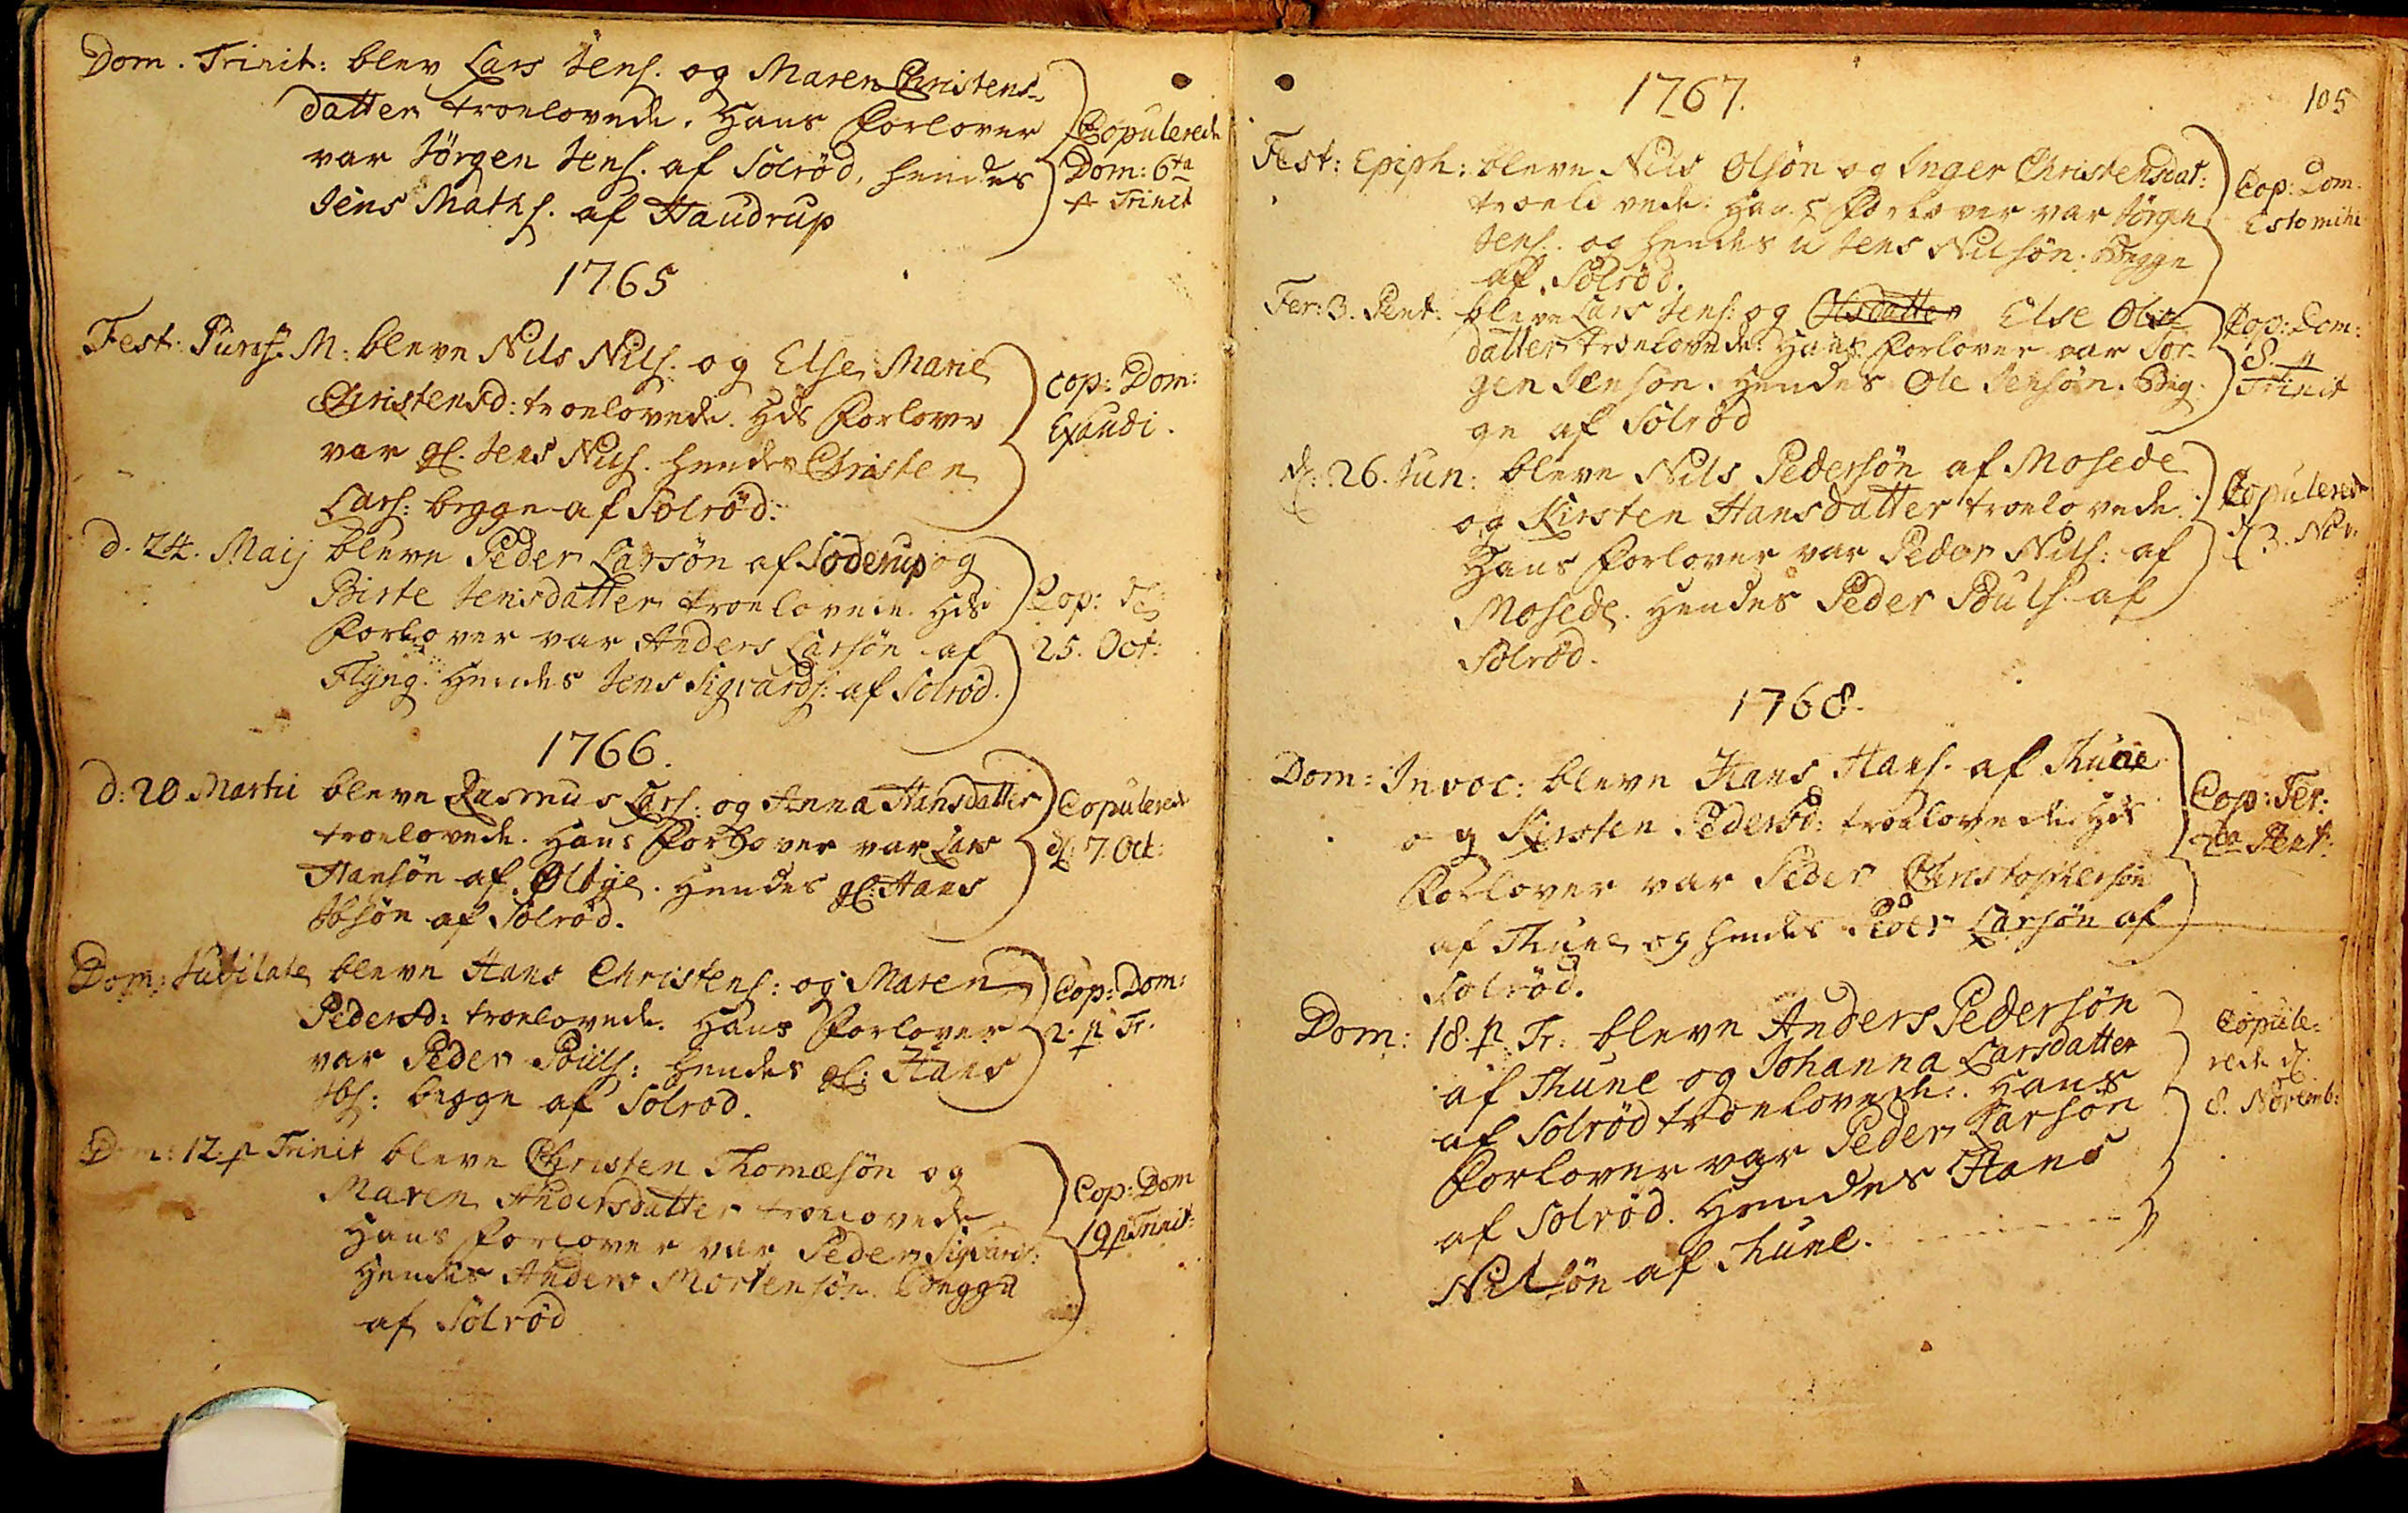Dom. Trinit: blev Lars Jens. og Maren Christens¬
datter troelovede. Hans Forlover
var Jørgen Jens. af Solrød, hendes
Jens Maths. af Haudrup
Copulerede
Dom: 6te
p Trinit
1765
Fest: Purif. M: bleve Nils Nils. og Else Marie
Christensd: troelovede. Hds Forlover
var gl. Jens Nils. hendes Christen
Lars: begge af Solrød.
Cop: Dom:
Exaudi.
d. 24. Maij bleve Peder larsøn af Soderup og
Birte Jensdatter troelovede. Hds
Forlover var Anders Larsøn af
Flyng. Hendes Jens Sigvards: af Solrød
Cop: d:
25. Oct:
1766.
d: 20 Martii bleve Rasmus Lars: og Anna Hansdatter
troelovede. Hans Forlover var Lars
Hansøn af Ølbye. Hendes gl: Hans
Ibsøn af Solrød.
Copulerede
d: 7. Oct:
Dom: Jubilate bleve Hans Christens: og Maren
Pedersd: troelovede. Hans Forlover
var Peder Pouls: hendes gl: Hans
Ibs: begge af Solrød.
Cop: Dom:
2. p Tr.
Dom: 12. p Trinit bleve Christen Thomæsøn og
Maren Andersdatter troelovede.
Hans Forlover var Peder Sigvards:
Hendes Anders Mortensøn. Begge
af Solrød.
Cop: Dom.
19 p Trinit
105
1767.
Fest: Epiph: bleve Nils Olsøn og Inger Christensdat:
troelovede. Hans Forlover var Jørgen
Jens. og hendes u Jens Nilsøn. Begge
af Solrød.
Cop: Dom:
Esto mihi
Fer: 3. Pent. bleve Lars Jens: og Olsdatter Else Ols¬
datter troelovede. Hans Forlover var Jør¬
gen Jensøn. Hendes Ole Jensøn. Beg¬
ge af Solrød
Cop: Dom:
8. p
Trinit
d: 26. Jun: bleve Nils Pedersøn af Mosede
og Kirsten Hansdatter troelovede
Hans Forlover var Peder Nils: af
Mosede. Hendes Peder Pouls. af
Solrød.
Copulerede
d 3. Nov:
1768.
Dom: Invoc: bleve Hans Hans. af Thune
og Kirsten Pedersd: troelovede Hds
Forlover var Peder Christophersøn
af Thune og hendes Peder Larsøn af
Solrød.
Cop: Fer:
2da Pent:
Dom: 18. p Tr: bleve Anders Pedersøn
af Thune og Johanna Larsdatter
af Solrød troelovede. Hans
Forlover var Peder Larsøn
af Solrød. Hendes Hans
Nilsøn af Thune.
Copule¬
rede d.
8. Novembr:
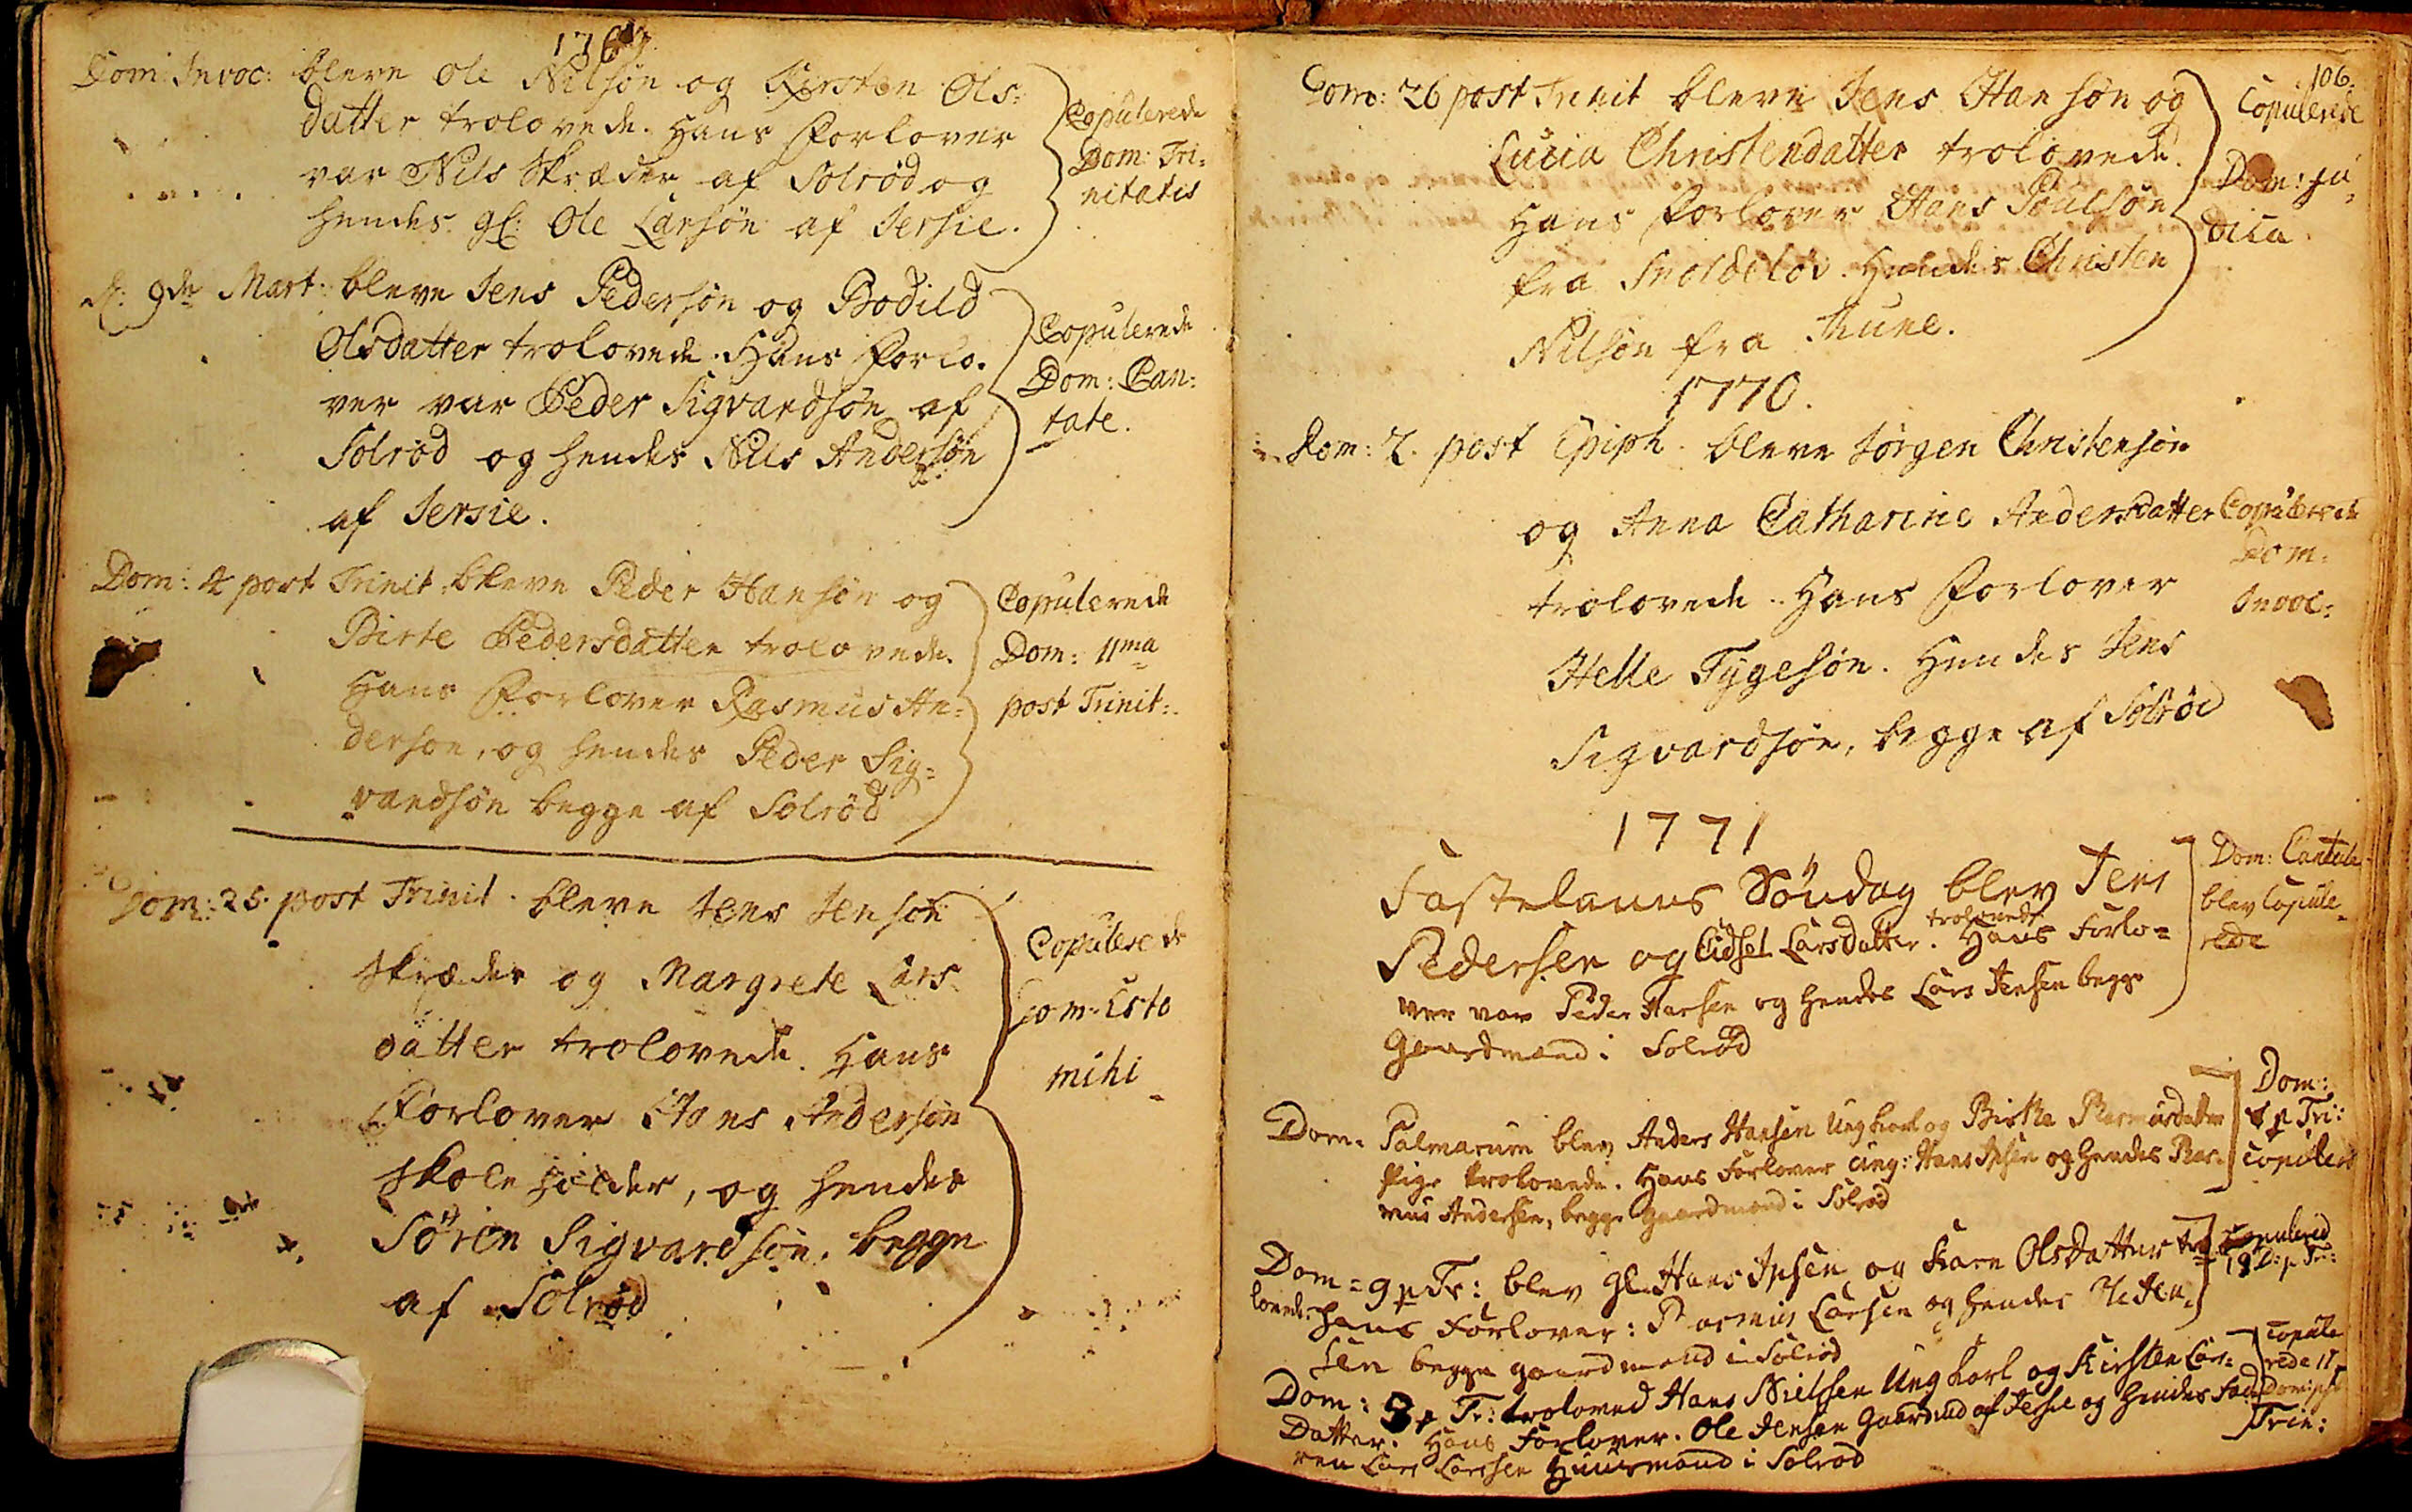1769.
Dom: Invoc: bleve Ole Nilsøn og Kirsten Ols¬
datter trolovede. Hans Forlover
var Nils Skræder af Solrød og
hendes gl: Ole Larsøn af Jersie.
Copulerede
Dom: Tri¬
nitatis
d: 9de Mart: bleve Jens Pedersøn og Bodild
Olsdatter trolovede. Hans Forlo¬
var var Peder Sigvardsøn af
Solrød og hendes Nils Andersøn
af Jersie.
Copulerede
Dom: Can:
tate.
Dom: 4 post Trinit: bleve Peder Hansøn og
Birte Pedersdatter trolovede.
Hans Forlover Rasmus An¬
dersøn, og hendes Peder Sig¬
vardsøn begge af Solrød
Copulerede
Dom: 11ma
post Trinit:
Dom: 25. post Trinit. bleve Jens Jensøn
Skræder og Margrete Lars¬
datter trolovede. Hans
Forlover. Hans Andersøn
Skoleholder, og hendes
Søren Sigvardsøn. begge
af Solrød
Copulerede
Dom: Esto
mihi
106.
Dom: 26 post Trinit bleve Jens Hansøn og
Lucia Christendatter trolovede.
Hans Forlover Hans Poulsøn
fra Snoldeløv. Hendes Christen
Nilsøn fra Thune.
Copulerede
Dom: Ju¬
dica.
1770.
Dom: 2. post Epiph. bleve Jørgen Christensøn
og Anna Catharine Andersdatter
trolovede. Hans Forlover
Helle Thygesøn. Hendes Jens
Sigvardsøn, begge af Solrød
Copulerede
Dom:
Invoc:
1771
Fastelauns Søndag blev Jens
trolovede
Pedersen og Cidsel Larsdatter. Hans Forlo¬
ver var Peder Hansen og hendes Lars Jensen begge
Gaardmænd i Solrød
Dom: Cantate
blev Copule¬
rede
Dom: Palmarum blev Anders Hansen Ungkarl og Birthe Rasmusdatter
Pige trolovede. Hans Forlover Ung: Hans Ipsen og hendes Ras¬
mus Andersen, begge Gaardmænd i Solrød
Dom:
7## p Tri:
Copulered
Dom: 9 p Tr: blev gl. Hans Ipsen og Karn Olsdatter tro¬
lovede. Hans Forlover: Rasmus Larsen og hendes Ole Jen¬
sen. begge Gaardmænd i Solrød
Copulered
12t: p Tr:
Dom: 8## p Tr: troloved Hans Nielssen Ungkarl og Kirsten Lars¬
Datter. Hans Forlover. Ole Jensen Gaardmd af Jersie og hendes Fade
ren Lars Larssen Huusmand i Solrød
Copule
rede 11##
Dom: pst
Trin: